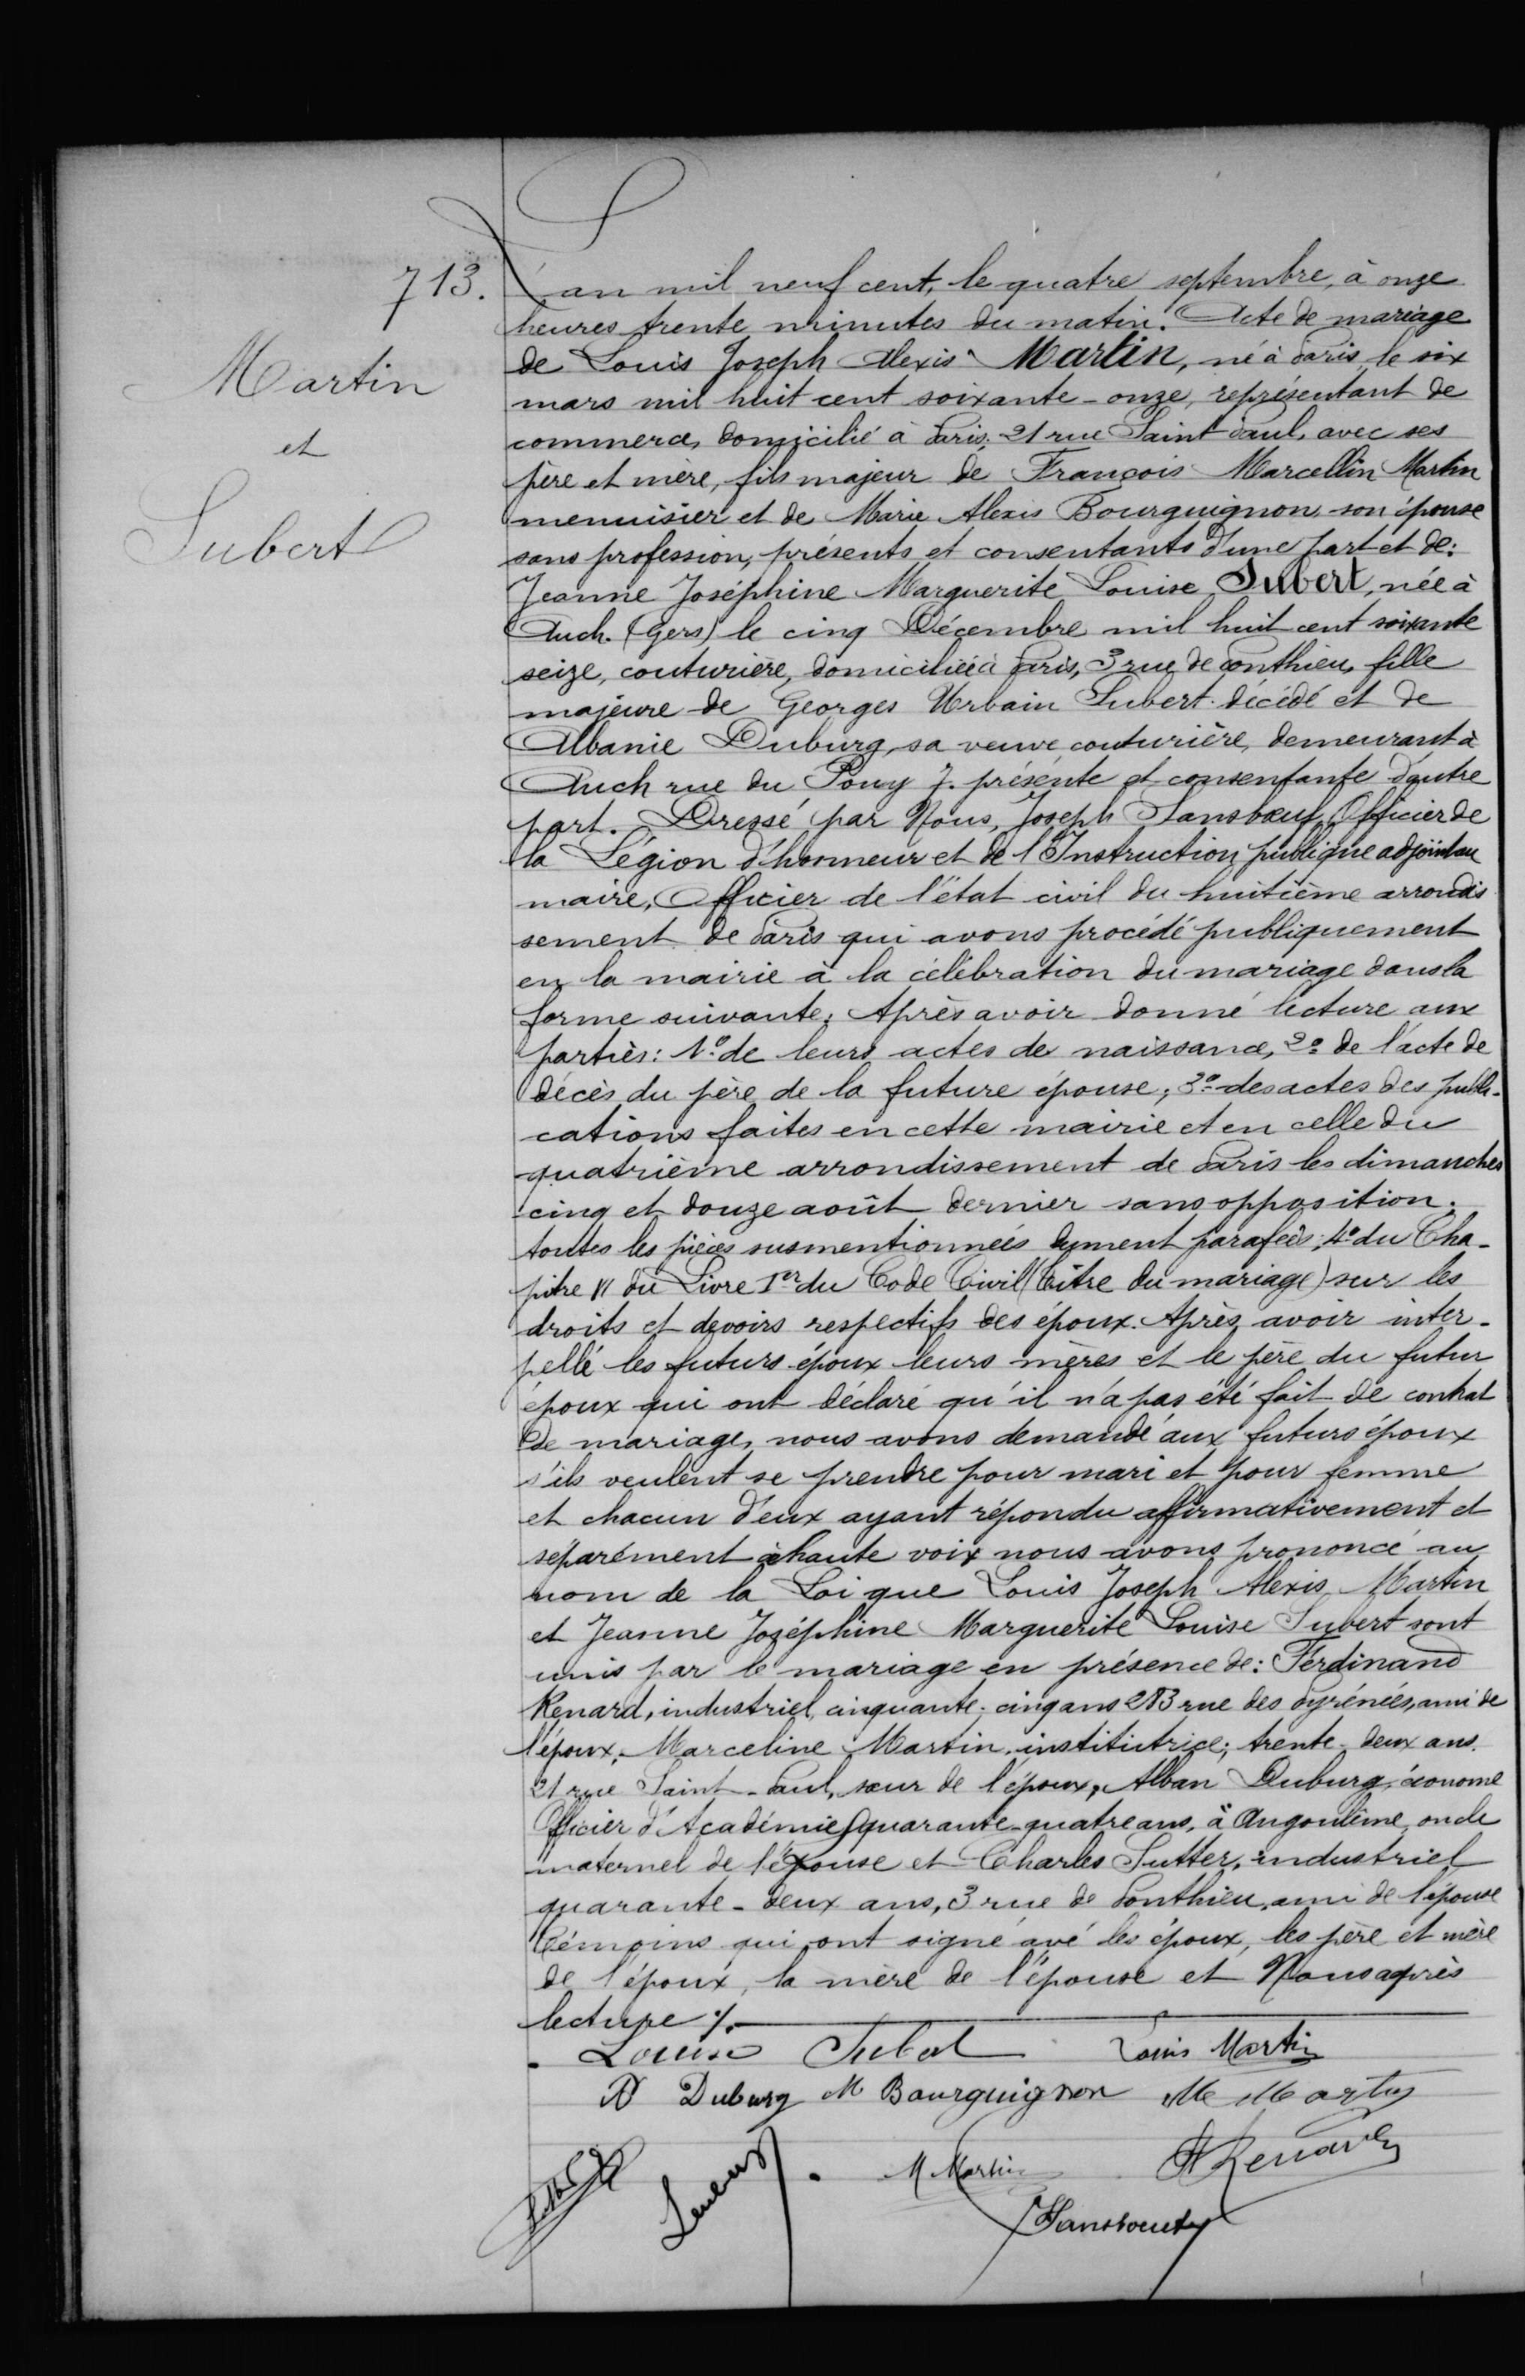Martin
et
Subert
L'an mil neuf cent, le quatre septembre, à onze
heures trente minutes du matin. Acte de mariage
de Louis Joseph Alexis Martin, né à Paris, le six
mars mil huit cent soixante-onze, représentant de
commerce, domicilié à Paris, 21 rue Saint Paul, avec ses
père et mère, fils majeure de François Marcellin Martin
menuisier et de Marie Alexis Bourguignons son épouse
sans profession, présents et consentants d'une part et de:
Jeanni Joséphine Marguerite Louise Subert, née à
Auch (Gers), le cinq Décembre mil huit cent soixante
seize, couturière domiciliée à Paris, 3 rue des conthieu, fille
majeure de Georges Urban Subert décédé et de
Albanie Duburg, sa veuve, couturière, demeurant)
Auch rue du Pony présente et consentante d'autre
part. Dressé par Nous, Joseph Sansbeuf, Officier de
la Légion d'Honneur et de l'Instruction publique adjointau
maire, Officier de l'état civil du huitième arrondis
semenet de Paris qui avons procédé publiquement
en la mairie à la célébration du mariage dans la
forme suivant: Après avoir donné lecture aux
parties: 1° de leurs actes de naissance, 2° de l'acte de
décès du père de la future épouse; 3° des actes des publi
cations faites en cette mairie et en celle du
quatrième arrondissement de Paris le dimanches
cinq et douze août dernier sans oppositione:
toutes les pièces susmentionnées dument parafées 4° du Cha-
pitre VI du Livre Ier du Code Civil (Titre du mariage) sur les
droits et devoirs respectifs des époux. Après avoir inter-
pellé les futurs époux leurs mères et le père du futur
époux qui ont déclaré qu'il n'a pas été fait de contrat
de mariage, nous avons demandé aux futurs époux
s'ils veulent se prendre pour mari et poru femme
et chacun d'eux ayant répondu affirmativement et
séparement à haute voix nous avons prononcé au
nom de la Loi que Louis Joseph Alexis Martin
et Jeanne Joséphine Marguerite Louise Subert sont
unis par le mariage en présence de: Ferdinand
Renard, industrier, cinquante-cinq ans 13 rue des Pyrénées, ami de
l'époux; Marceline Martin institutrice, trente deux ans
et rue Saint-Paul, soeur de l'époux, Alban Duburg, économe,
Officier d'Académie quarante quatre ans, à Angoulème, oncle
maternel de l'épouse et Charles Sutter, industriel
quarante-deux ans, 3 rue de Ponthieu, ami de l'épouse
témoins qui ont signé avec les époux, les père et mère
de l'époux, la mère de l'épouse et Nous après
lecture ./.
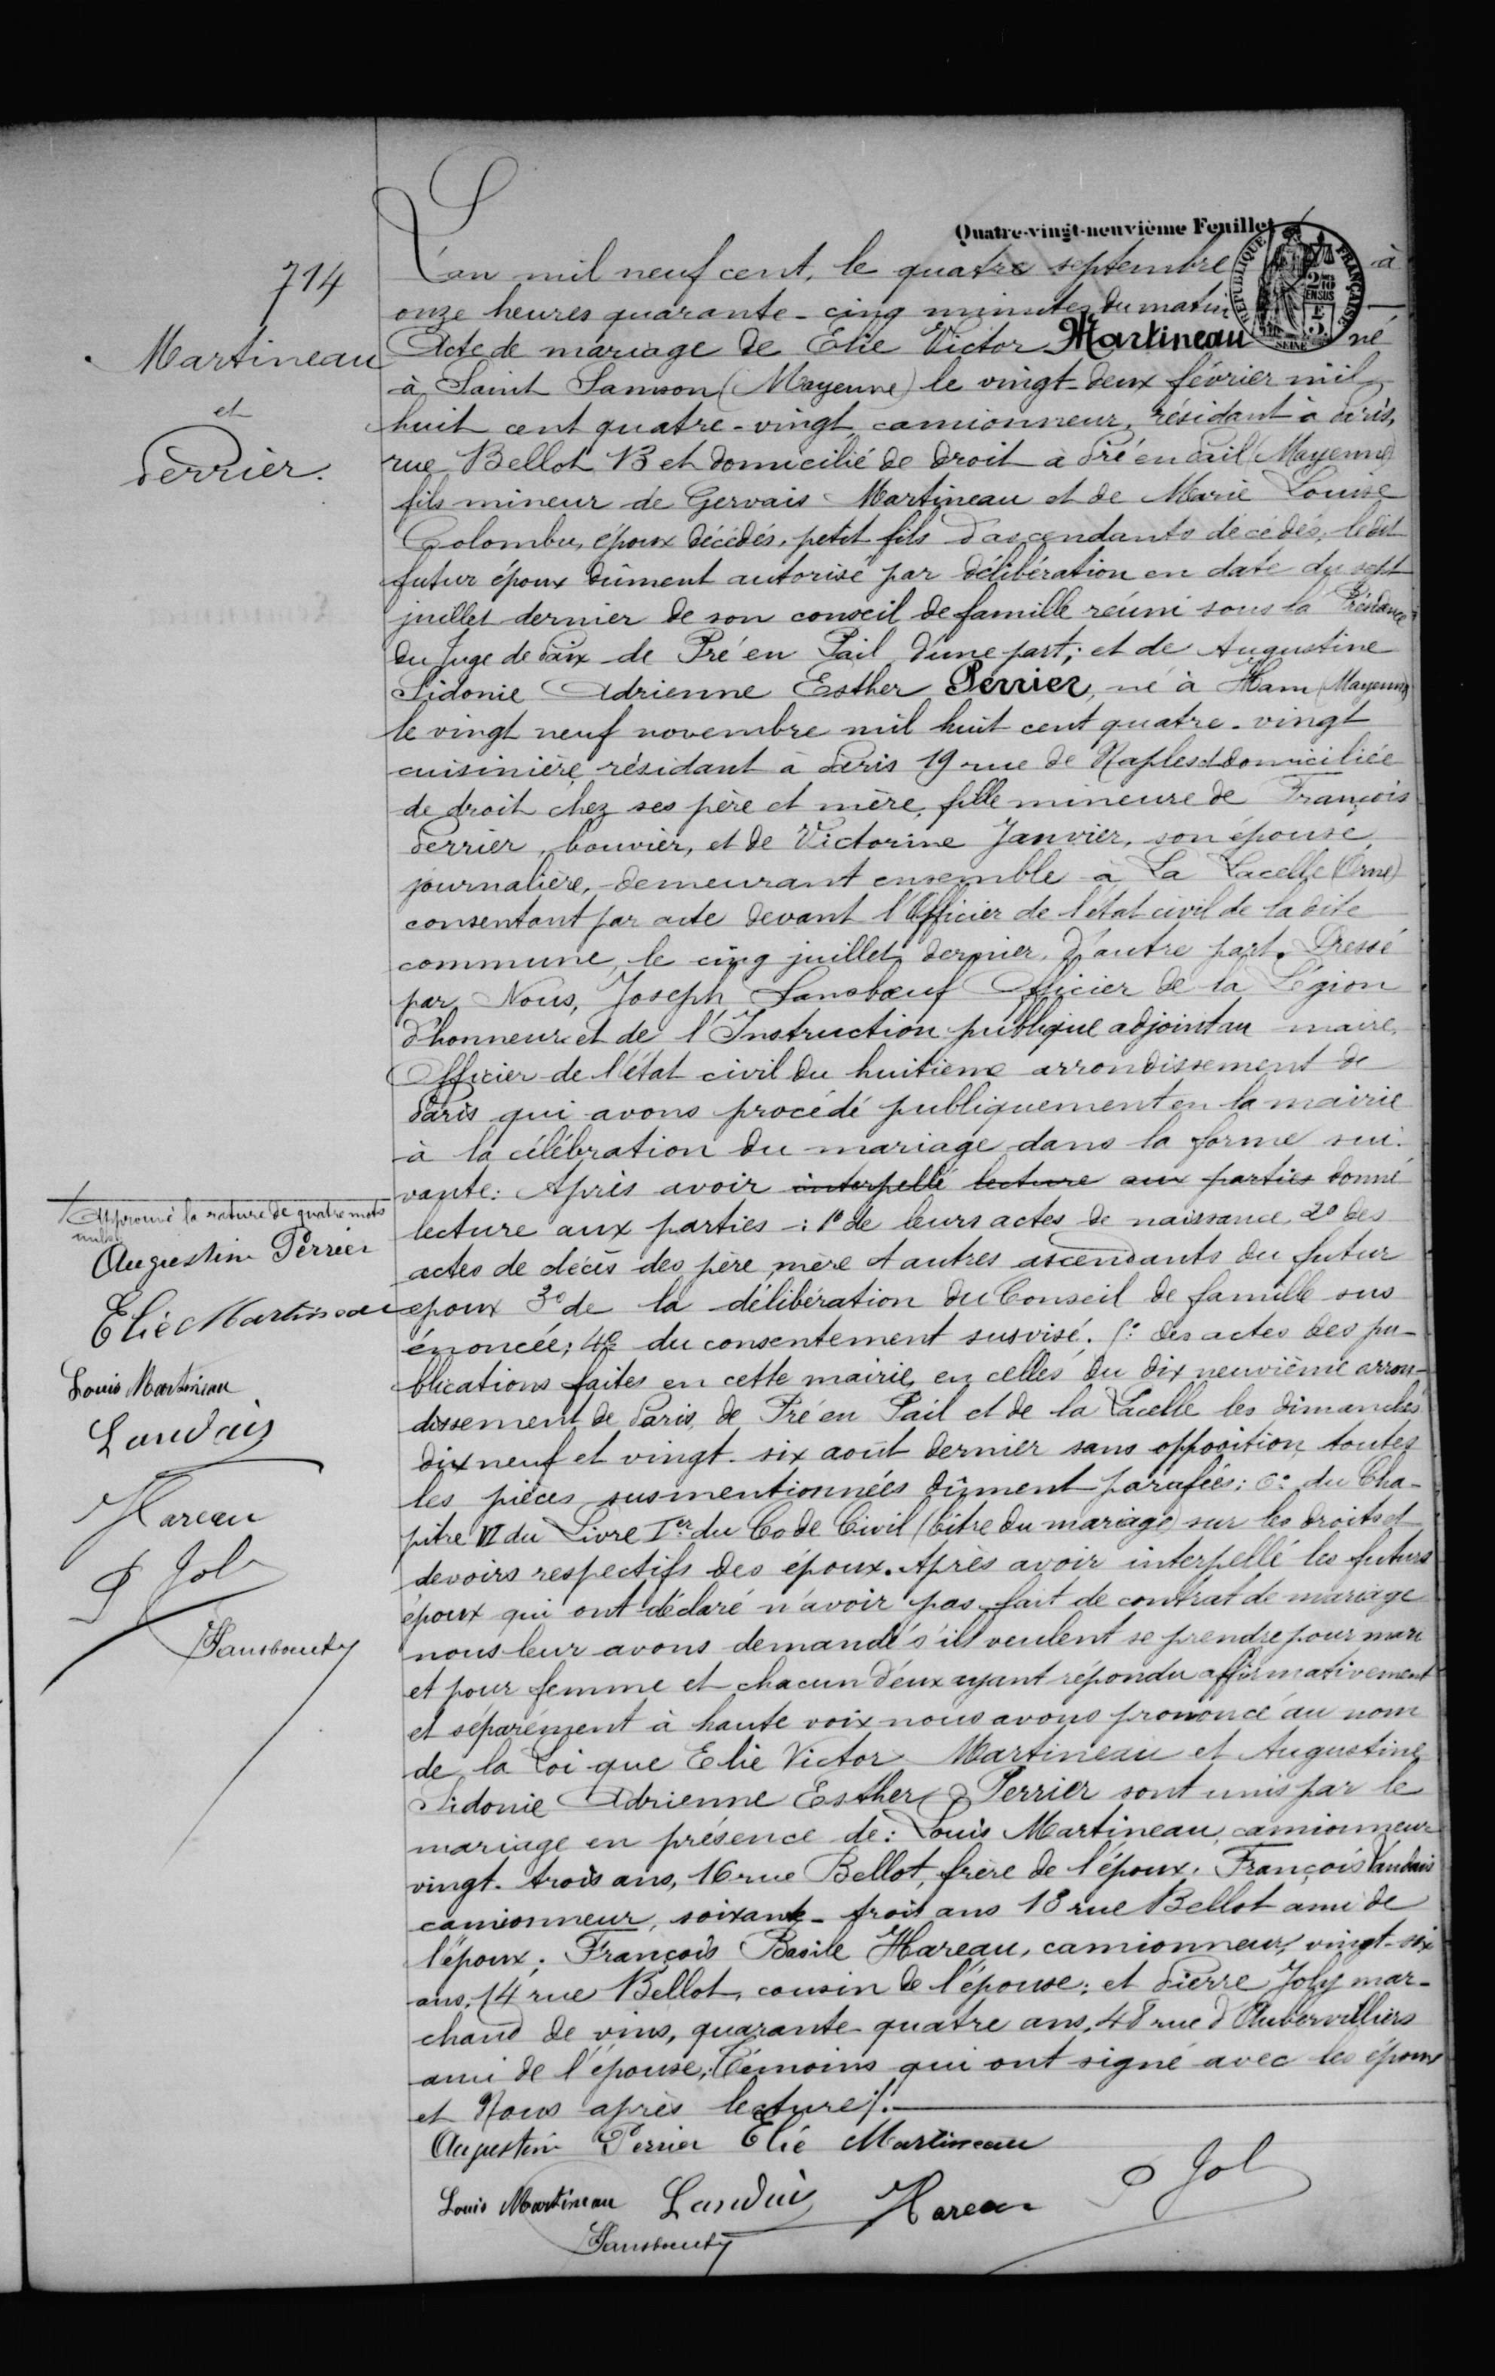714
Martineau
et
Perrier
L'an mil neuf cent, le quatre septembre à
onze heures quarante-cinq minutes le matin -
Acte de mariage de Elie Victor Martineau né
à Saint Samon (Mayenne) le vingt-deux février mil
huit cent quatre-vingt camionneur, résidant à Paris
rue Bellot 13 et domicilié le droit à Préendail (Mayenne)
fils mineur de Gervais Martineau et de Marie Louise
Colombu, époux décédés, petit fils d'ascendants décédés, le dit
futur époux dûment autorisé par délibération en date du sept
juillet dernier de son conseil de famille réussi sous la Présidence
du Juge de Paix de Pré en Pail d'une part; et de Augustine
Sidonie Adrienne Esther Perrier, né à Ham (Mayenne)
le vingt neuf novembre mil huit cent quatre-vingt
cuisinière résidant à Paris 19 rue de Naples domiciliée
de droit chez ses père et mère fille mineure de François
Perrier, bouvier, et de Victorine Janvier, son épouse
journalière, demeurant ensemble à la Lacelle (Orne)
consentant par acte devant l'Officier de l'état civil de la dite
commune, le cinq juillet dernier, d'autre part. Dressé
par Nous, Joseph Sanoboeuf Officier de la Légion
d'honneur et de l'Instruction publique adjoint au maire,
Officier de l'état civil du huitième arrondissement de
Paris, qui avons procédé publiquement en la mairie
à la célébration du mariage dans l aforme sui
vant. Après avoir donné
lecture aux parties: 1° de leurs actes de naissance 2° des
actes de décès des père mère et autres ascendants du futur
epoux 3° de la délibération du Conseil de famille sus
énoncée; 4° du consentement susvisé; 5° des actes des pu-
blications faites en cette mairie et en celle du dix neuvième arron-
dissement de Paris de Pré en Pail et de la Lacelle les dimanches
dix neuf et vingt-six août dernier sans opposition, toutes
les pièces susmentionnées dûment parafées; 6° du Cha-
pitre VI du Livre Ier du Code Civil (Titre du mariage) sur les droits et
devoirs respectifs des époux. Après avoir interpellé les futurs
époux qui ont déclaré n'avoir pas fait de contrat de mariage
nous leur avons demandé s'ils veulent se prendre pour mari
et pour femme et chacun d'eux ayant répondu affirmativement
et séparèment à haute voix nous avons prononcé au nom
de la Loi que Elie Victor Martineau et Augustine
Sidonie Adrienne Esther Perrier sont unis par le
mariage en présence de: Louis Martineau, camionneur
vingt-trois ans, 16 rue Bellot frère de l'époux, François Vaudois
camionneur, soixante-trois ans 18 rue Bellot ami de
l'époux; François Basile Hareau, camionneur, vingt-six
ans, 14 rue Bellot, cousin de l'épouse, et Pierre Joly mar-
chand de vingt, quarante quatre ans, 48 rue d'Aubervilliers
ami de l'épouse, Témoins qui ont signé avec les époux
et Nous après lecture./.-